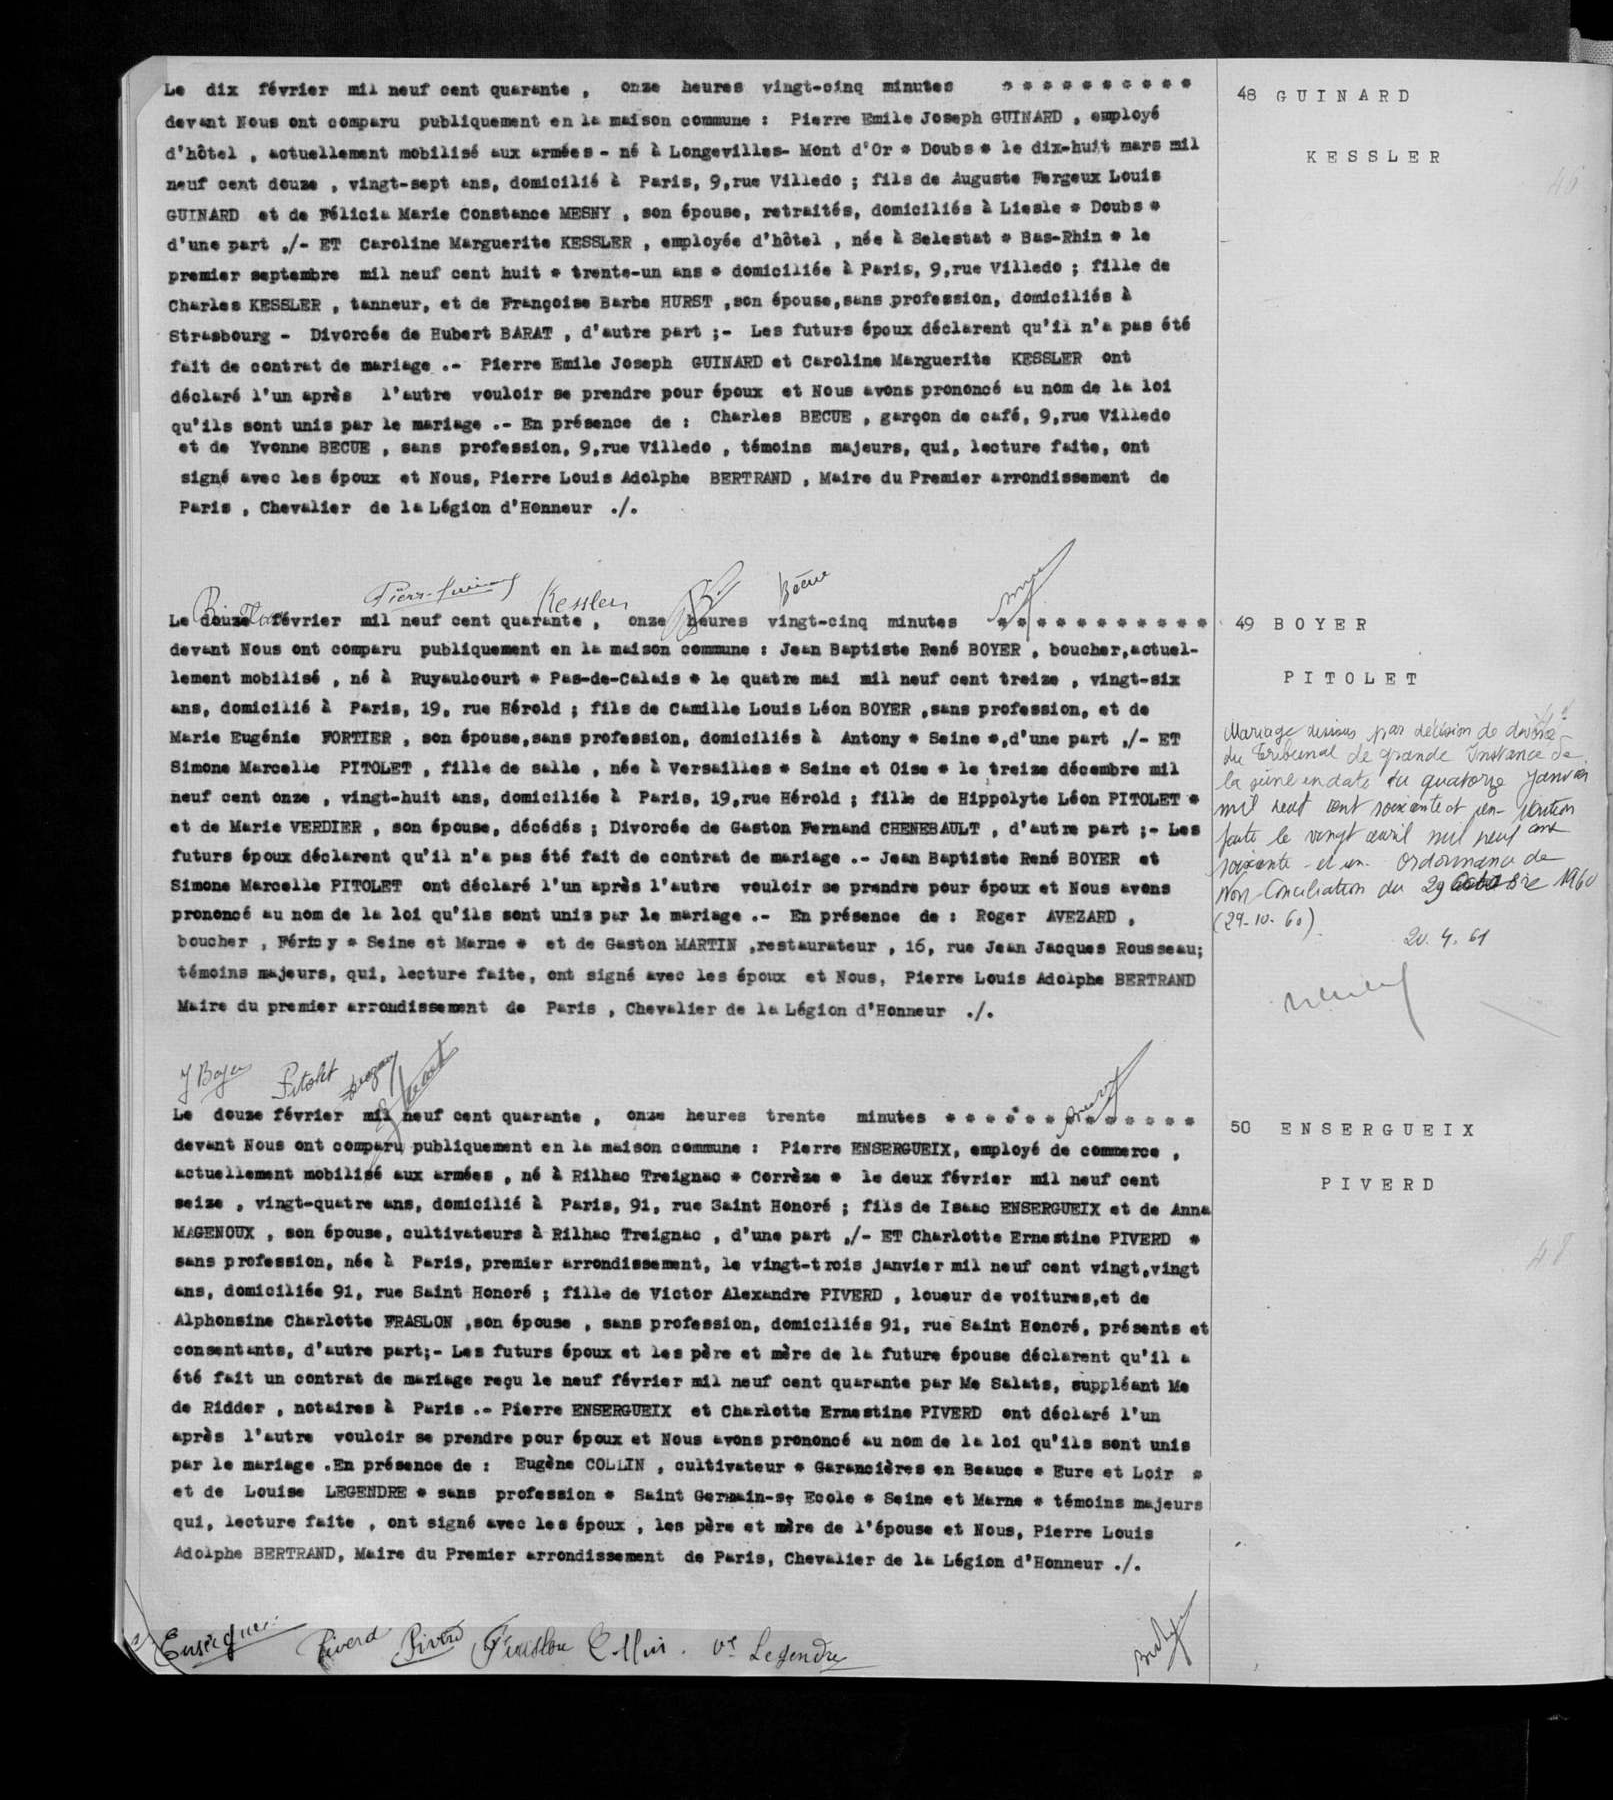Le dix février mil neuf cent quarante, onze heures vingt-cinq minutes ****
devant Nous ont comparu publiquement en la maison commune: Pierre Emile Joseph GUINARD, employé
d'hôtel, actuellement mobilisé aux armées-né à Longevilles-Mont d'Or * Doubs * le dix-huit mars mil
neuf cent douze, vingt-sept ans, domicilié à Paris, 9, rue Villede; fils de Auguste Fergeux Louis
GUINARD et de Félicia Marie Constance MESNY, son épouse, retraités, domiciliés à Liesle * Doubs *
d'une part ,/-ET Caroline Marguerite KESSLER, employée d'hôtel, née à Selestat * Bas-Rhin * le
premier septembre mil neuf cent huit * trente-un ans * domiciliée à Paris, 9, rue Villedo; fille de
Charles KESSLER, tanneur, et de Françoise Barbe HURST, son épouse, sans profession, domiciliés à
Strasbourg-Divorcée de Hubert BARAT, d'autre part ;-Le futurs époux déclarent qu'il n'a pas été
fait de contrat de mariage .-Pierre Emile Joseph GUINARD et Caroline Marguerite KESSLER ont
déclaré l'un après l'autre vouloir se prendre pour époux et Nous avons prononcé au nom de la loi
qu'ils sont unis par le mariage .-En présence de: Charles BECUE, garçon de café, 9, rue Villedo
et de Yvonne BECUE, sans profession, 9, rue Villedo, témoins majeurs, qui, lecture faite, ont
signé avec les époux et Nous, Pierre Louis Adolphe BERTRAND, Maire du Premier arrondissement de
Paris, Chevalier de la Légion d'Honneur ./.
Le douze février mil neuf cent quarante, onze heure vingt-cinq minutes ****
devant Nous ont comparu publiquement en la maison commune: Jean Baptiste René BOYER, boucher, actuel-
lement mobilisé, né à Ruyaulcourt * Pas-de-Calais * le quatre mai mil neuf cent treize, vingt-six
ans, domicilié à Paris, 19, rue Hérold; fils de Camille Louis Léon BOYER, sans profession, et de
Marie Eugénie FORTIER, son épouse, sans profession, domiciliés à Antony * Seine *, d'une part ,/-ET
Simone Marcelle PITOLET, fille de salle, née à Versailles * Seine et Oise * le treize décembre mil
neuf cent onze, vingt-huit ans, domiciliée à Paris, 19, rue Hérold; fille de Hippolyte Léon PITOLET *
et de Marie VERDIER, son épouse, décédés; Divorcée de Gaston Fernand CHENEBAULT, d'autre part ;-Les
futurs époux déclarent qu'il n'a pas été fait de contrat de mariage .-Jean Baptiste René BOYER et
Simone Marcelle PITOLET ont déclaré l'un après l'autre vouloir se prendre pour époux et Nous avons
prononcé au nom de la loi qu'ils sont unis par le mariage .-En présence de: Roger AVEZARD,
boucher, Féricy * Seine et Marne * et de Gaston MARTIN, restaurateur, 16, rue Jean Jacques Rousseau;
témoins majeurs, qui, lecture faite, ont signé avec les époux et Nous, Pierre Louis Adolphe BERTRAND
Maire du premier arrondissement de Paris, Chevalier de la Légion d'Honneur ./.
Le douze février mil neuf cent quarante, onze heures trente minutes ****
devant Nous ont comparu publiquement en la maison commune: Pierre ENSERGUEIX, employé de commerce,
actuellement mobilisé aux armées, né à Rilhac Treignac * Corrèze * le deux février mil neuf cent
seize, vingt-quatre ans, domicilié à Paris, 91, rue Saint Honoré; fils de Isaac ENSERGUEIX et de Anna
MAGENOUX, son épouse, cultivateurs à Rilhac Treignac, d'une part ,/-ET Charlotte Ernestine PIVERD *
sans profession, née à Paris, premier arrondissement, le vingt-trois janvier mil neuf cent vingt, vingt
ans, domicilié 91, rue Saint Honoré; fille de Victor Alexandre PIVERD, loueur de voitures, et de
Alphonsine Charlotte FRASLON, son épouse, sans profession, domiciliés 91, rue Saint Honoré, présents et
consentants, d'autre part ;-Les futurs époux et les père et mère de la future épouse déclarent qu'il a
été fait un contrat de mariage reçu le neuf février mil neuf cent quarante par Me Salats, suppléant Me
de Ridder, notaires à Paris .-Pierre ENSERGUEIX et Charlotte Ernestine PIVERD ont déclaré l'un
après l'autre vouloir se prendre pour époux et Nous avons prononcé au nom de la loi qu'ils sont unis
par le mariage. En présence de: Eugène COLLIN, cultivateur * Garancières en Beauce * Eure et Loir *
et de Louise LEGENDRE * sans profession * Saint Germain-St Ecole * Seine et Marne * témoins majeurs
qui, lecture faite, ont signé avec les époux, les père et mère de l'épouse et Nous, Pierre Louis
Adolphe BERTRAND, Maire du Premier arrondissement de Paris, Chevalier de la Légion d'Honneur ./.
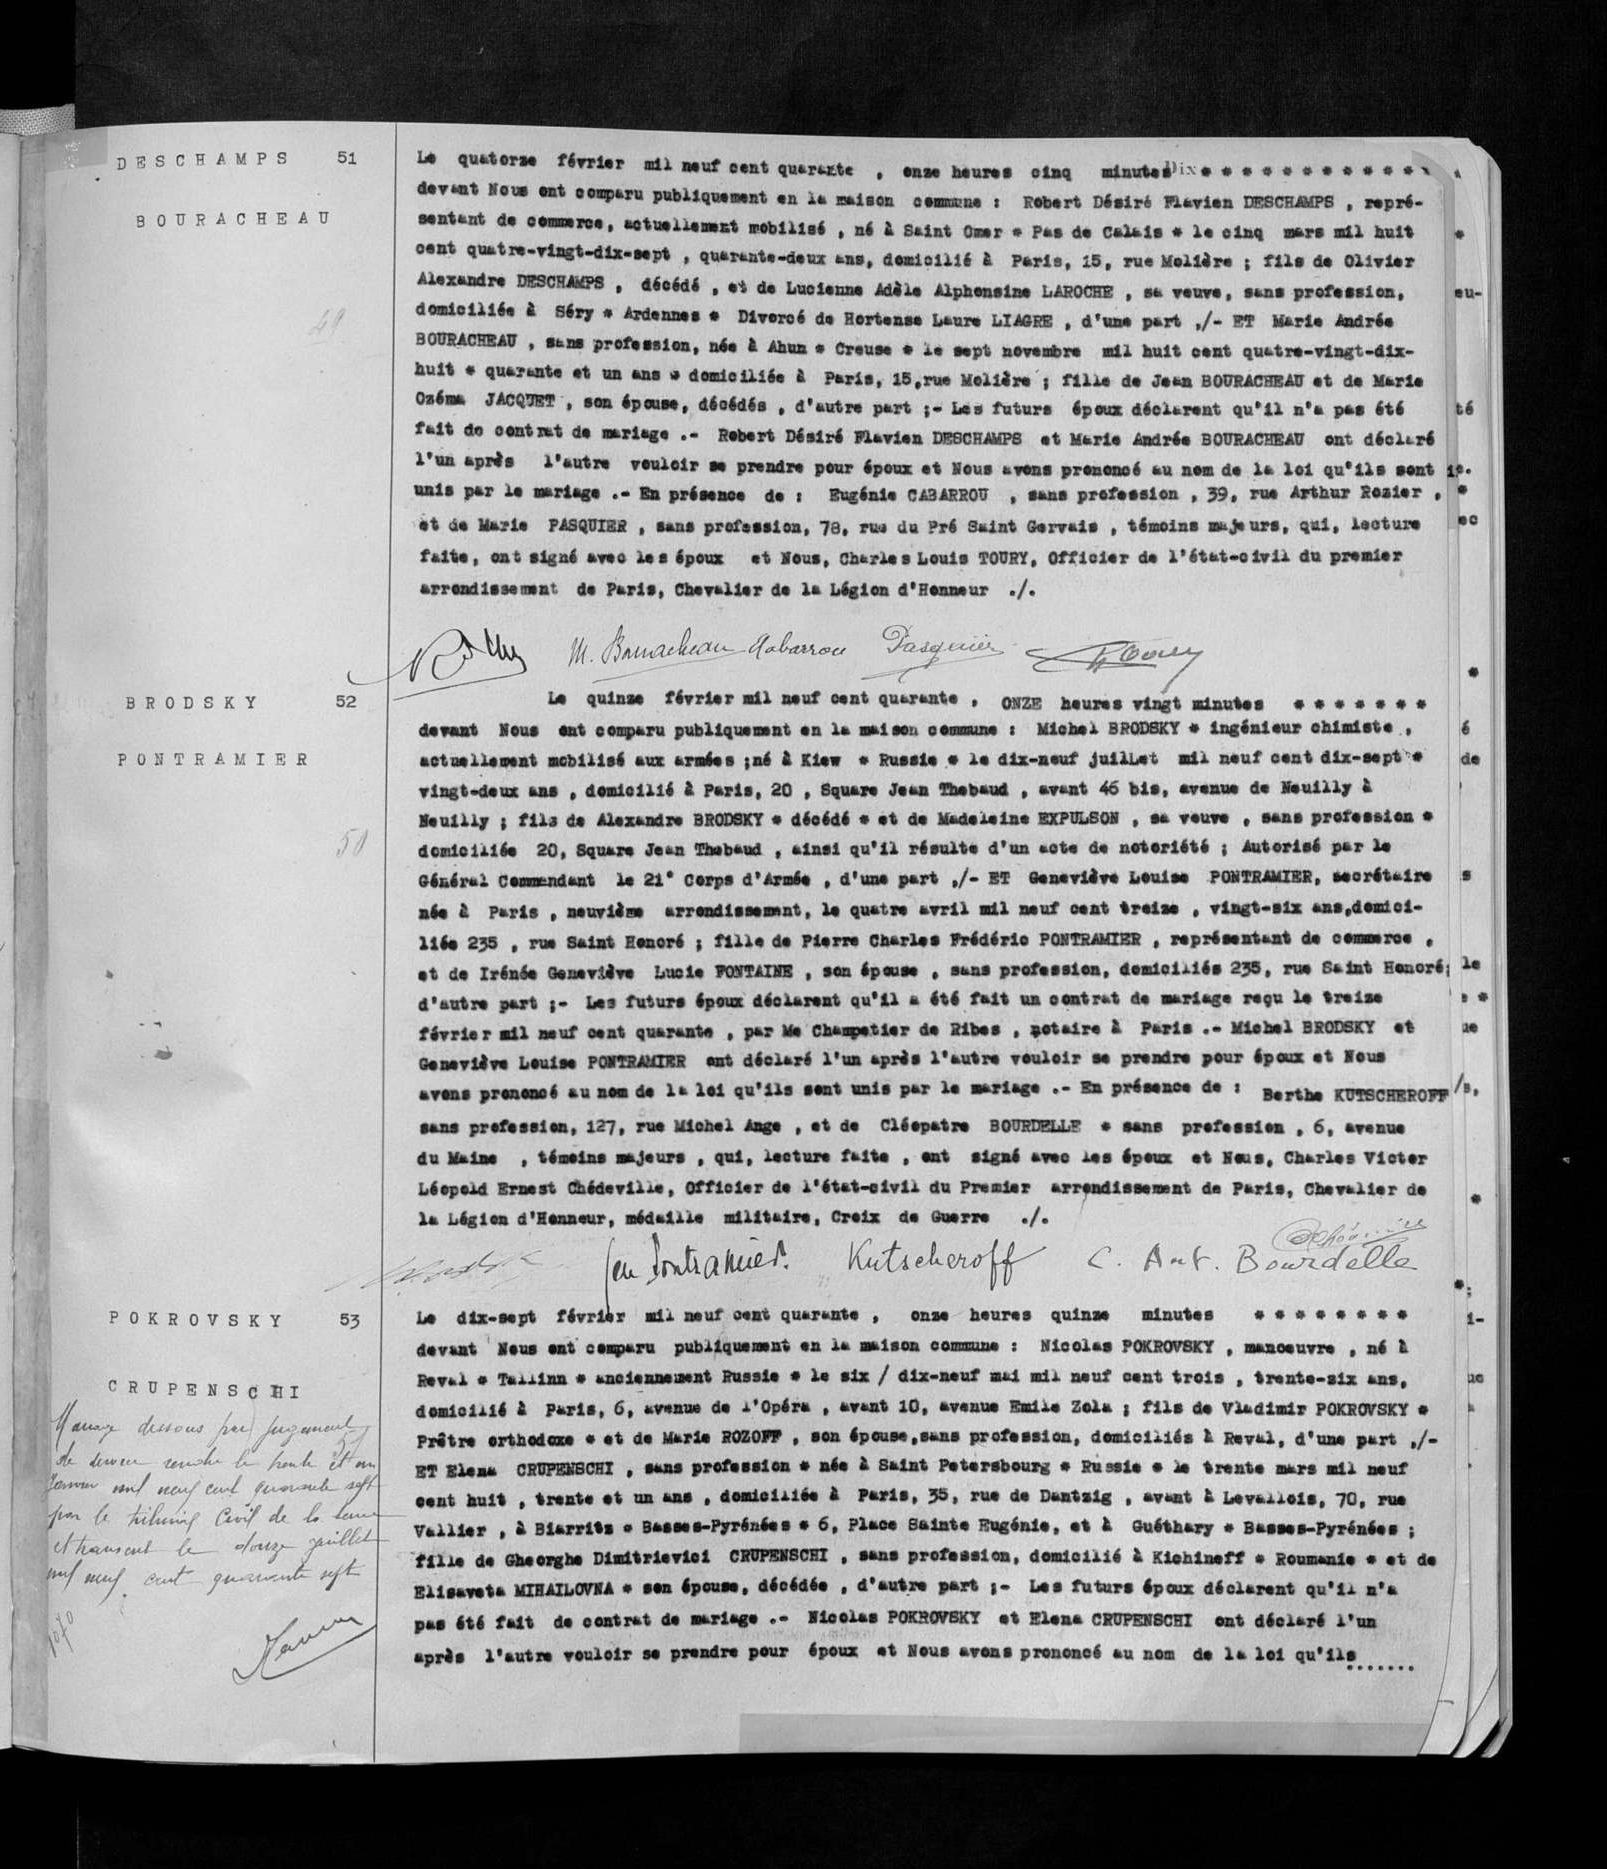Le quatorze février mil neuf cent quarante, onze heures cinq minutes ****
devant Nous ont comparu publiquement en la maison commune: Robert Désiré Flavien DESCHAMPS, repré-
sentant de commece, actuellement mobilisé, né à Saint Omer * Pas de Calais * le cinq mars mil huit
cent quatre-vingt-dix-sept, quarante-deux ans, domicilié à Paris, 15, rue Molière; fils de Olivier
Alexandre DESCHAMPS, décédé, et de Lucienne Adèle Alphonsine LAROCHE, sa veuve, sans profession,
domiciliée à Séry * Ardennes * Divorcé de Hortense Laure LIAGRE, d'une part ,/-ET Marie Andrée
BOURACHEAU, sans profession, née à Ahun * Creuse * le sept novembre mil huit cent quatre-vingt-dix-
huit * quarante et un ans * domiciliée à Paris, 15, rue Molière; fille de Jean BOURACHEAU et de Marie
Ozéma JACQUET, son épouse, décédés, d'autre part ;-Les futurs époux déclarent qu'il n'a pas été
fait de contrat de mariage .-Robert Désiré Flavien DESCHAMPS et Marie Andrée BOURACHEAU ont déclaré
l'un après l'autre vouloir se prendre pour époux et Nous avons prononcé au nom de la loi qu'ils sont
unis par le mariage .-En présence de: Eugénie CABARROU, sans profession, 39, rue Arthur Rozier,
et de Marie PASQUIER, sans profession, 78, rue du Pré Saint Gervais, témoins majeurs, qui, lecture
faite, ont signé avec les époux et Nous, Charles Louis TOURY, Officier de l'état-civil du premier
arrondissement de Paris, Chevalier de la Légion d'Honneur ./.
Le quinze février mil neuf cent quarante, ONZE heures vingt minutes ****
devant Nous ont comparu publiquement en la maison commune: Michel BRODKY * ingénieur chimiste,
actuellement mobilisé aux armées; né à Kiev * Russie * le dix-neuf juillet mil neuf cent dix-sept *
vingt-deux ans, domicilié à Paris, 20, Square jean Thebaud, avant 46 bis, avenue de Neuilly à
Neuilly; fils de Alexandre BRODSKY * décédé * et de Madeleine EXPULSON, sa veuve, sans profession *
domicilié 20, Square Jean Thebaud, ainsi qu'il résulte d'un acte de notoriété; Autorisé par le
Général Commendant le 21° Corps d'Armée, d'une part ,/-ET Geneviève Louise PONTRAMIER, sécrétaire
née à Paris, neuvième arrondissement, le quatre avril mil neuf cent treize, vingt-six ans, domici-
liée 235, rue Saint Honoré; fille de Pierre Charles Frédéric PONTRAMIER, représentant de commerce,
et de Irénée Geneviève Lucie FONTAINE, son épouse, sans profession, domiciliés 235, rue Saint Honoré
d'autre part ;-Les futurs époux déclarent qu'il a été fait un contrat de mariage reçu le treize
février mil neuf cent quarante, par Me Champetier de Ribes, notaire à Paris .-Michel BRODSKY et
Geneviève Louise PONTRAMIER ont déclaré l'un après l'autre vouloir se prendre pour époux et Nous
avons prononcé au nom de la loi qu'ils sont unis par le mariage .-En présence de: Berthe KUTSCHEROFF
sans profession, 127, rue Michel Ange, et de Cléopatre BOURDELLE * sans profession, 6, avenue
du Maine, témoins majeurs, qui, lecture faite, ont signé avec les époux et Nous, Charles Victor
Léopold Ernest Chédeville, Officier de l'état-civil du Premier arrondissement de Paris, Chevalier de
la Légion d'Honneur, médailler militaire, Croix de Guerre ./.
Le dix-sept février mil neuf cent quarante, onze heures quinze minutes ****
devant Nous ont comparu publiquement en la maison commune: Nicolas POKROVSKY, manoeuvre, né à
Reval * Tallinn * anciennement Russie * le six/ dix-neuf mai mil neuf cent trois, trente-six ans,
domicilié à Paris, 6, avenue de l'Opéra, avant 10, avenue Emile Zola; fils de Valdimir POKROVSKY *
Prêtre orthodoxe * et de Marie ROZOFF, son épouse, sans profession, domiciliés à Reval, d'une part ,/
Et Elena CRUPENSCHI, sans profession * née à Saint Petersbourg * Russie * le trente mars mil neuf
cent huit, trente et un ans, domiciliée à Paris, 35, rue de Dantzig, avant à Levallois, 70, rue
Vallier, à Biarritz * Basses-Pyrénées * 6, Place Sainte Eugénie, et à Guéthary * Basses-Pyrénées;
fille de Gheorghe Dimitrievici CRUPENSCHI, sans profession, domicilié à Kichineff * Roumanie * et de
Elisaveta MIHAILOVNA* son épouse, décédée, d'autre part ;-Les futurs époux déclarent qu'il n'a
pas été fait de contrat de mariage .-Nicolas POKROVSKY et Elena CRUPENSCHI ont déclaré l'un
après l'autre vouloir se prendre pour époux et Nous avons prononcé au nom de la loi qu'ils